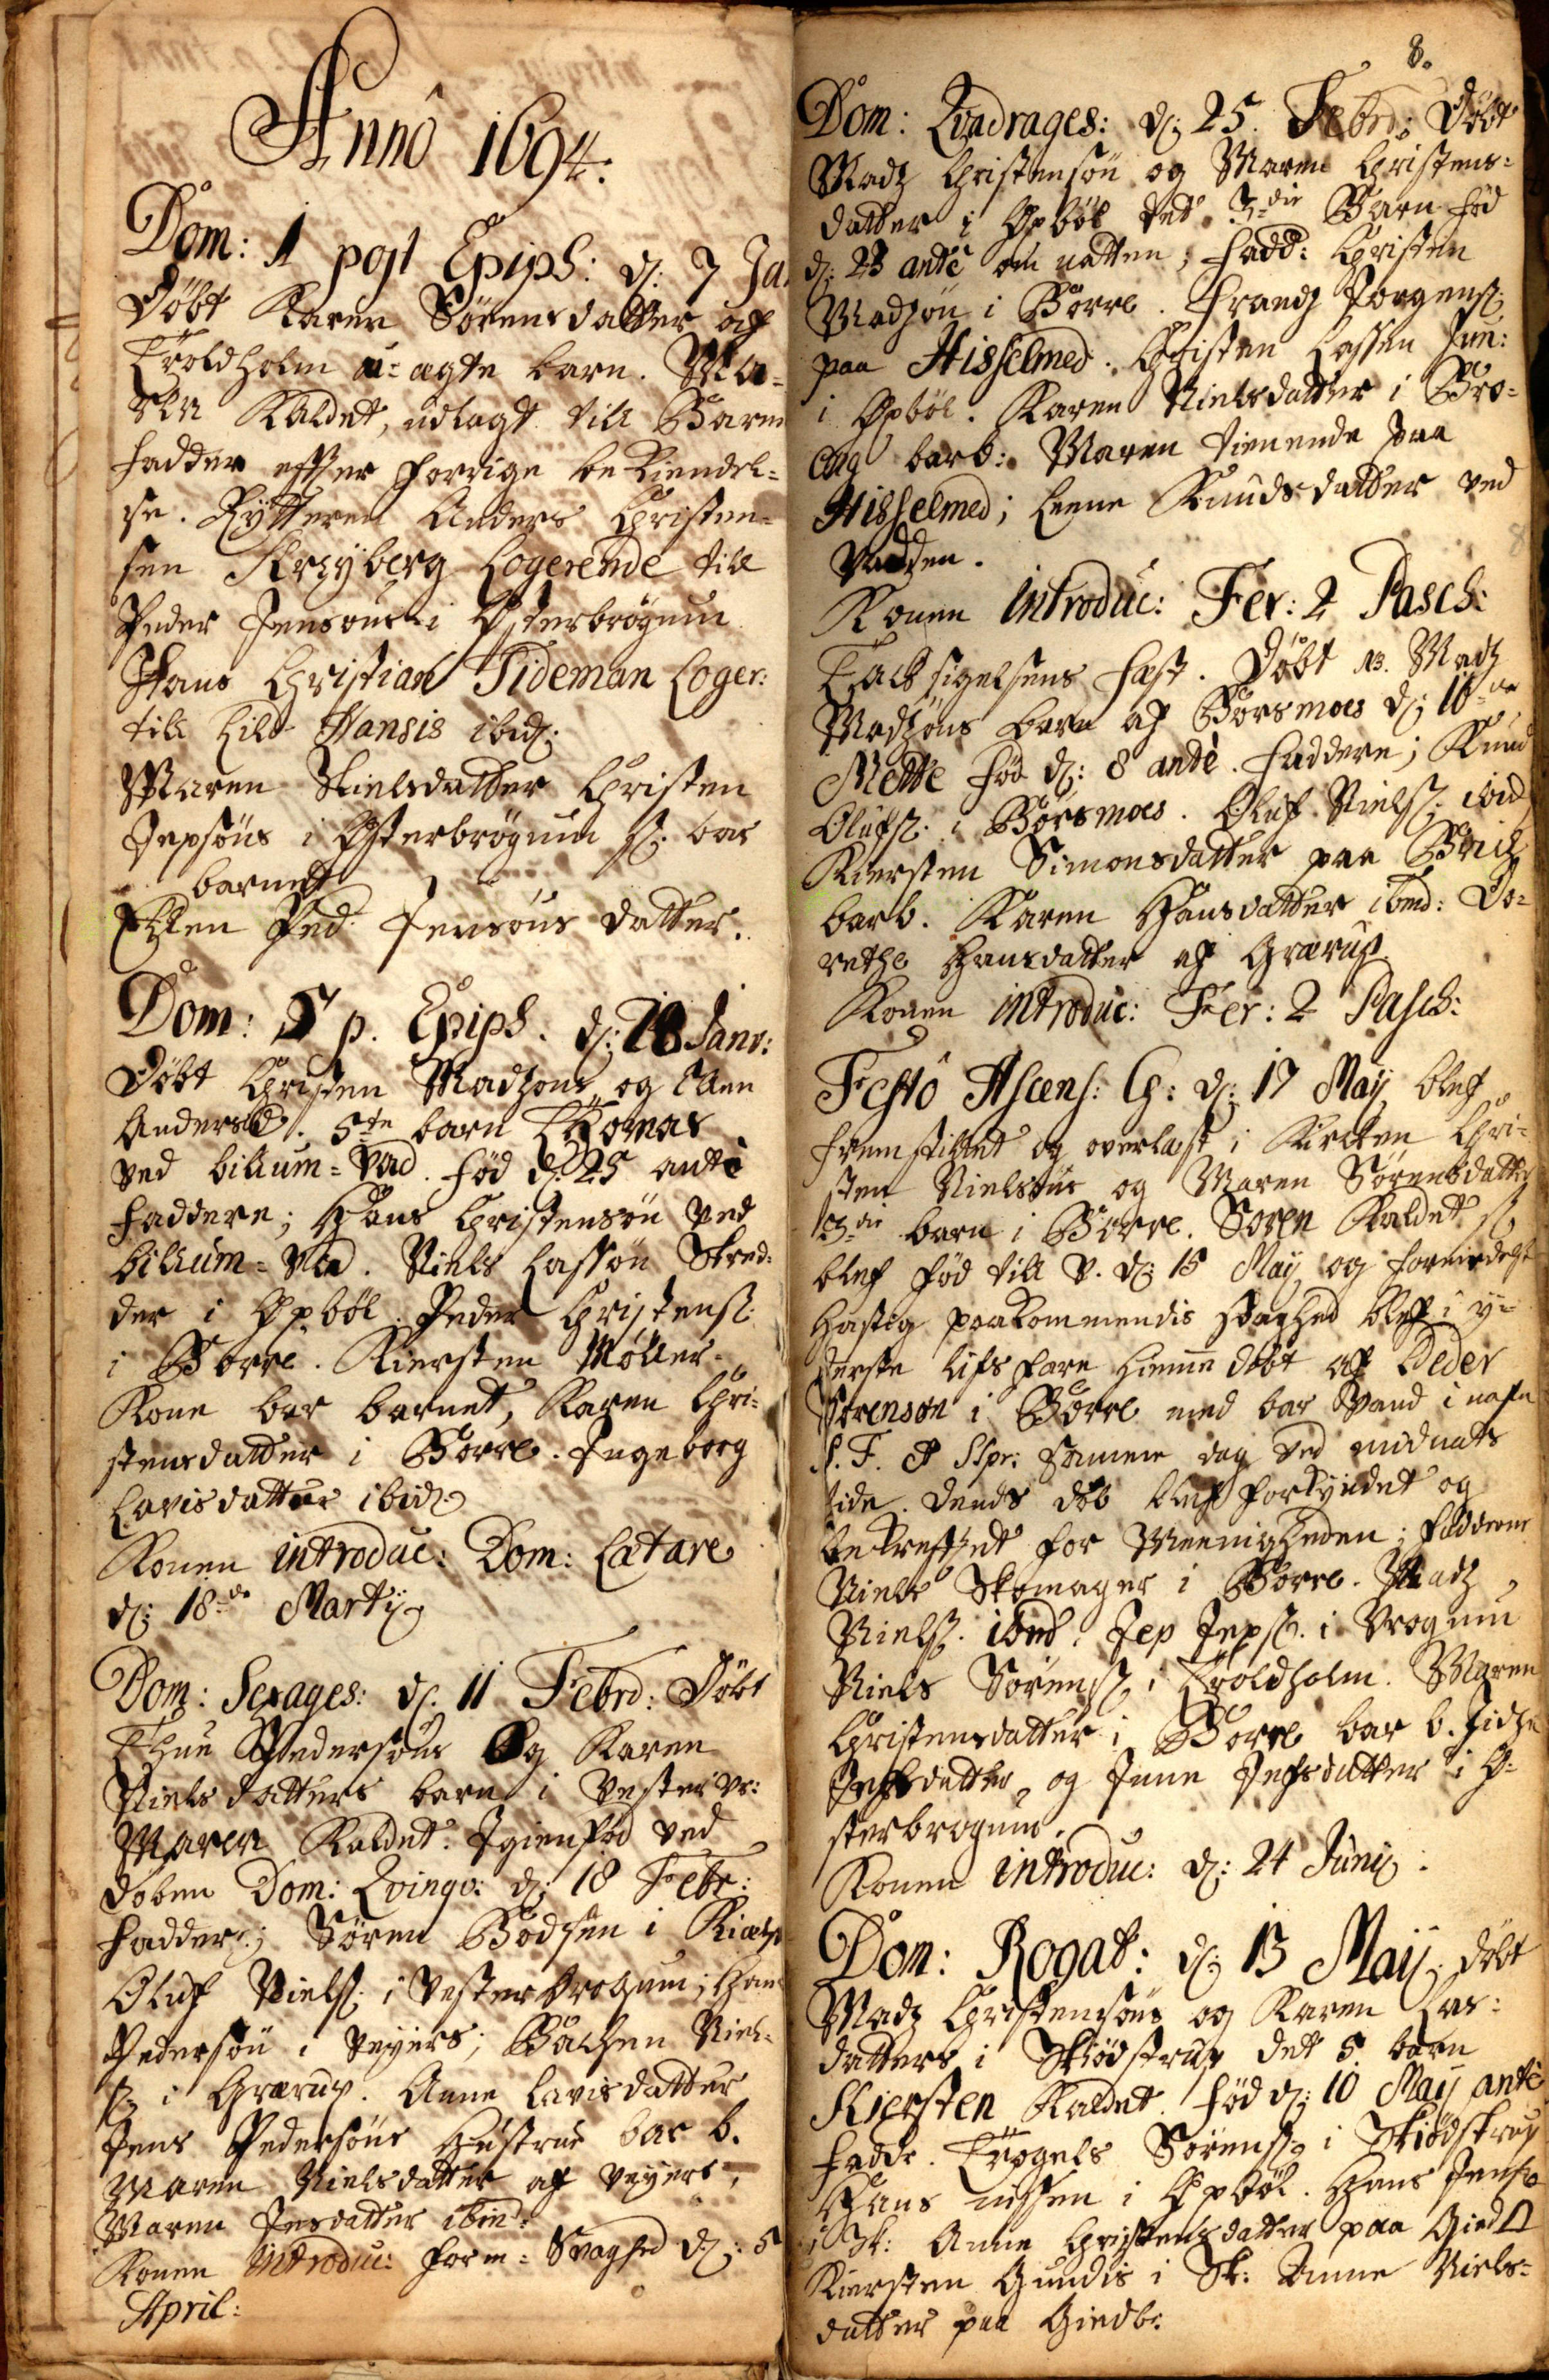Anno 1694:
Dom: 1 post Epiph: d: 7 Ja
Døbt Karen Sørens datter af
Troldholm u-ægte barn. Ma¬
ren kaldet, udlagt till Barne¬
Faddere effter forrige bekiendel¬
se. Rytteren Anders Christen¬
sen Kryberg Logerende till
Peder Jensøns i Østerbrøgum.
Hans Christian Tideman Loger:
til Lild Hansis ibid:
Maren Nielsdatter Christen
Jepsøns i Østerbrøgum s. bar
Barnet.
Ellen Ped: Jensøns datter.
Dom: 5 p. Epiph. d: 28 Janv:
Døbt Christen Madzøns og Ellen
Andersd. 5te barn Thomas
ved billum=vad fød d. 25 ante
Faddere; Hans Christensøn ved
billum=vad, Niels Lassøn Skred¬
der i Oxbøl, Peder Christens.
i Borre. Kiersten Møller¬
Kone bar barnet, Karen Chri¬
stensdatter i Borre . Ingeborg
Lavisdatter ibid.
Konen introduc: Dom: Latare
d: 18de Martij.
Dom: Sexages: d. 11 Febr: Døbt
Thue Pedersøns og Karen
Niels datters barn i Vester Vr:
Maren Kaldet: Igienfød ved
doben Dom: Qvinqv: d: 18 Febr:
Faddere: Søren Bodsen i Kiæ##
Oluf Niels: i Vester brogum; Hans
Pedersøn i Veijers; Bachen Niel¬
s i Grærup. Anne Lavisdatter
Jens Pedersøns Hustrue bar b.
Maren Nielsdatter af Veyers,
Maren Jesdatter ibid:
Konen introduc: for m=Svaghed d: 5
April:
8
Dom. Qvadrages: d 25. Febr: Døbt
Madz Christensøn og Maren Christens¬
datter i Oxbøl det 3die Barn fød
d: 23 ante om natten; Fadd: Christen
Madzøn i Borre, Frandz Jorgens.
paa Hisselmed. Christen Lassen Jun:
i Oxbøl. Karen Nielsdatter i Bro¬
eng barb: Maren tienende paa
Hisselmed; Leene Knudsdatter ved
Vadden.
Konen introduc: Fer. 2 Pasch:
Tacksigelsens Fæst. Døbt ##. Madz
Madzøns barn af Børsmoes d: 10de
Mette fød d: 8 ante. Faddere; Knud
Olufs. i Børsmoes. Oluf Niels. ibid.
Kiersten Simonsdatter paa Brich
barb. Karen Hansdatter ibm: Do¬
rethe Hansdatter af Grærup.
Konen introduc: Fer: 2 Pasch:
Festo Ascens: Ch: d: 17 May blef
fremstillet og overlæst i Kirchen Chri¬
sten Nielsøns og Maren Sørensdatters
3die Barn i Borre. Soren kaldet s
blef fød till V. d: 15 May og formiedelst
hastig paakommendis Svaghed blef i y¬
derste lifs fare hiemme døbt af Peder
Sørensøn i Borre med bar Vand i nafn
S.F. ## Sspr: samme dag ved midnats
tide. dends dob blef forkyndet og
bekreftet for Meenigheden; Fadderne
Niels Skomager i Borre, Madz
Niels. ibm. Jep Jeps. i Vrogum
Niels Sørens i Troldholm. Maren
Christensdatter i Borre bar b. Zidze
Jepsdatter og Jene Jepsdatter i Ø¬
sterbrogum.
Konen introduc: d: 24 Junij.
Dom: Rogat: d:13 Maij døbt
Madz Christensøns og Karen Las¬
datters i Skiødstrup det 5. barn
Kiersten kaldet. fød d: 10 Maij ante
Faddr. Trogels Sørens i Skiødstrup
Hans ##sen i Oxbøl. Hans Jens
i Sk: Anne Christensdatter paa Gied##
Kiersten Gundis i Sk: Anne Niels¬
datter paa Giedb.
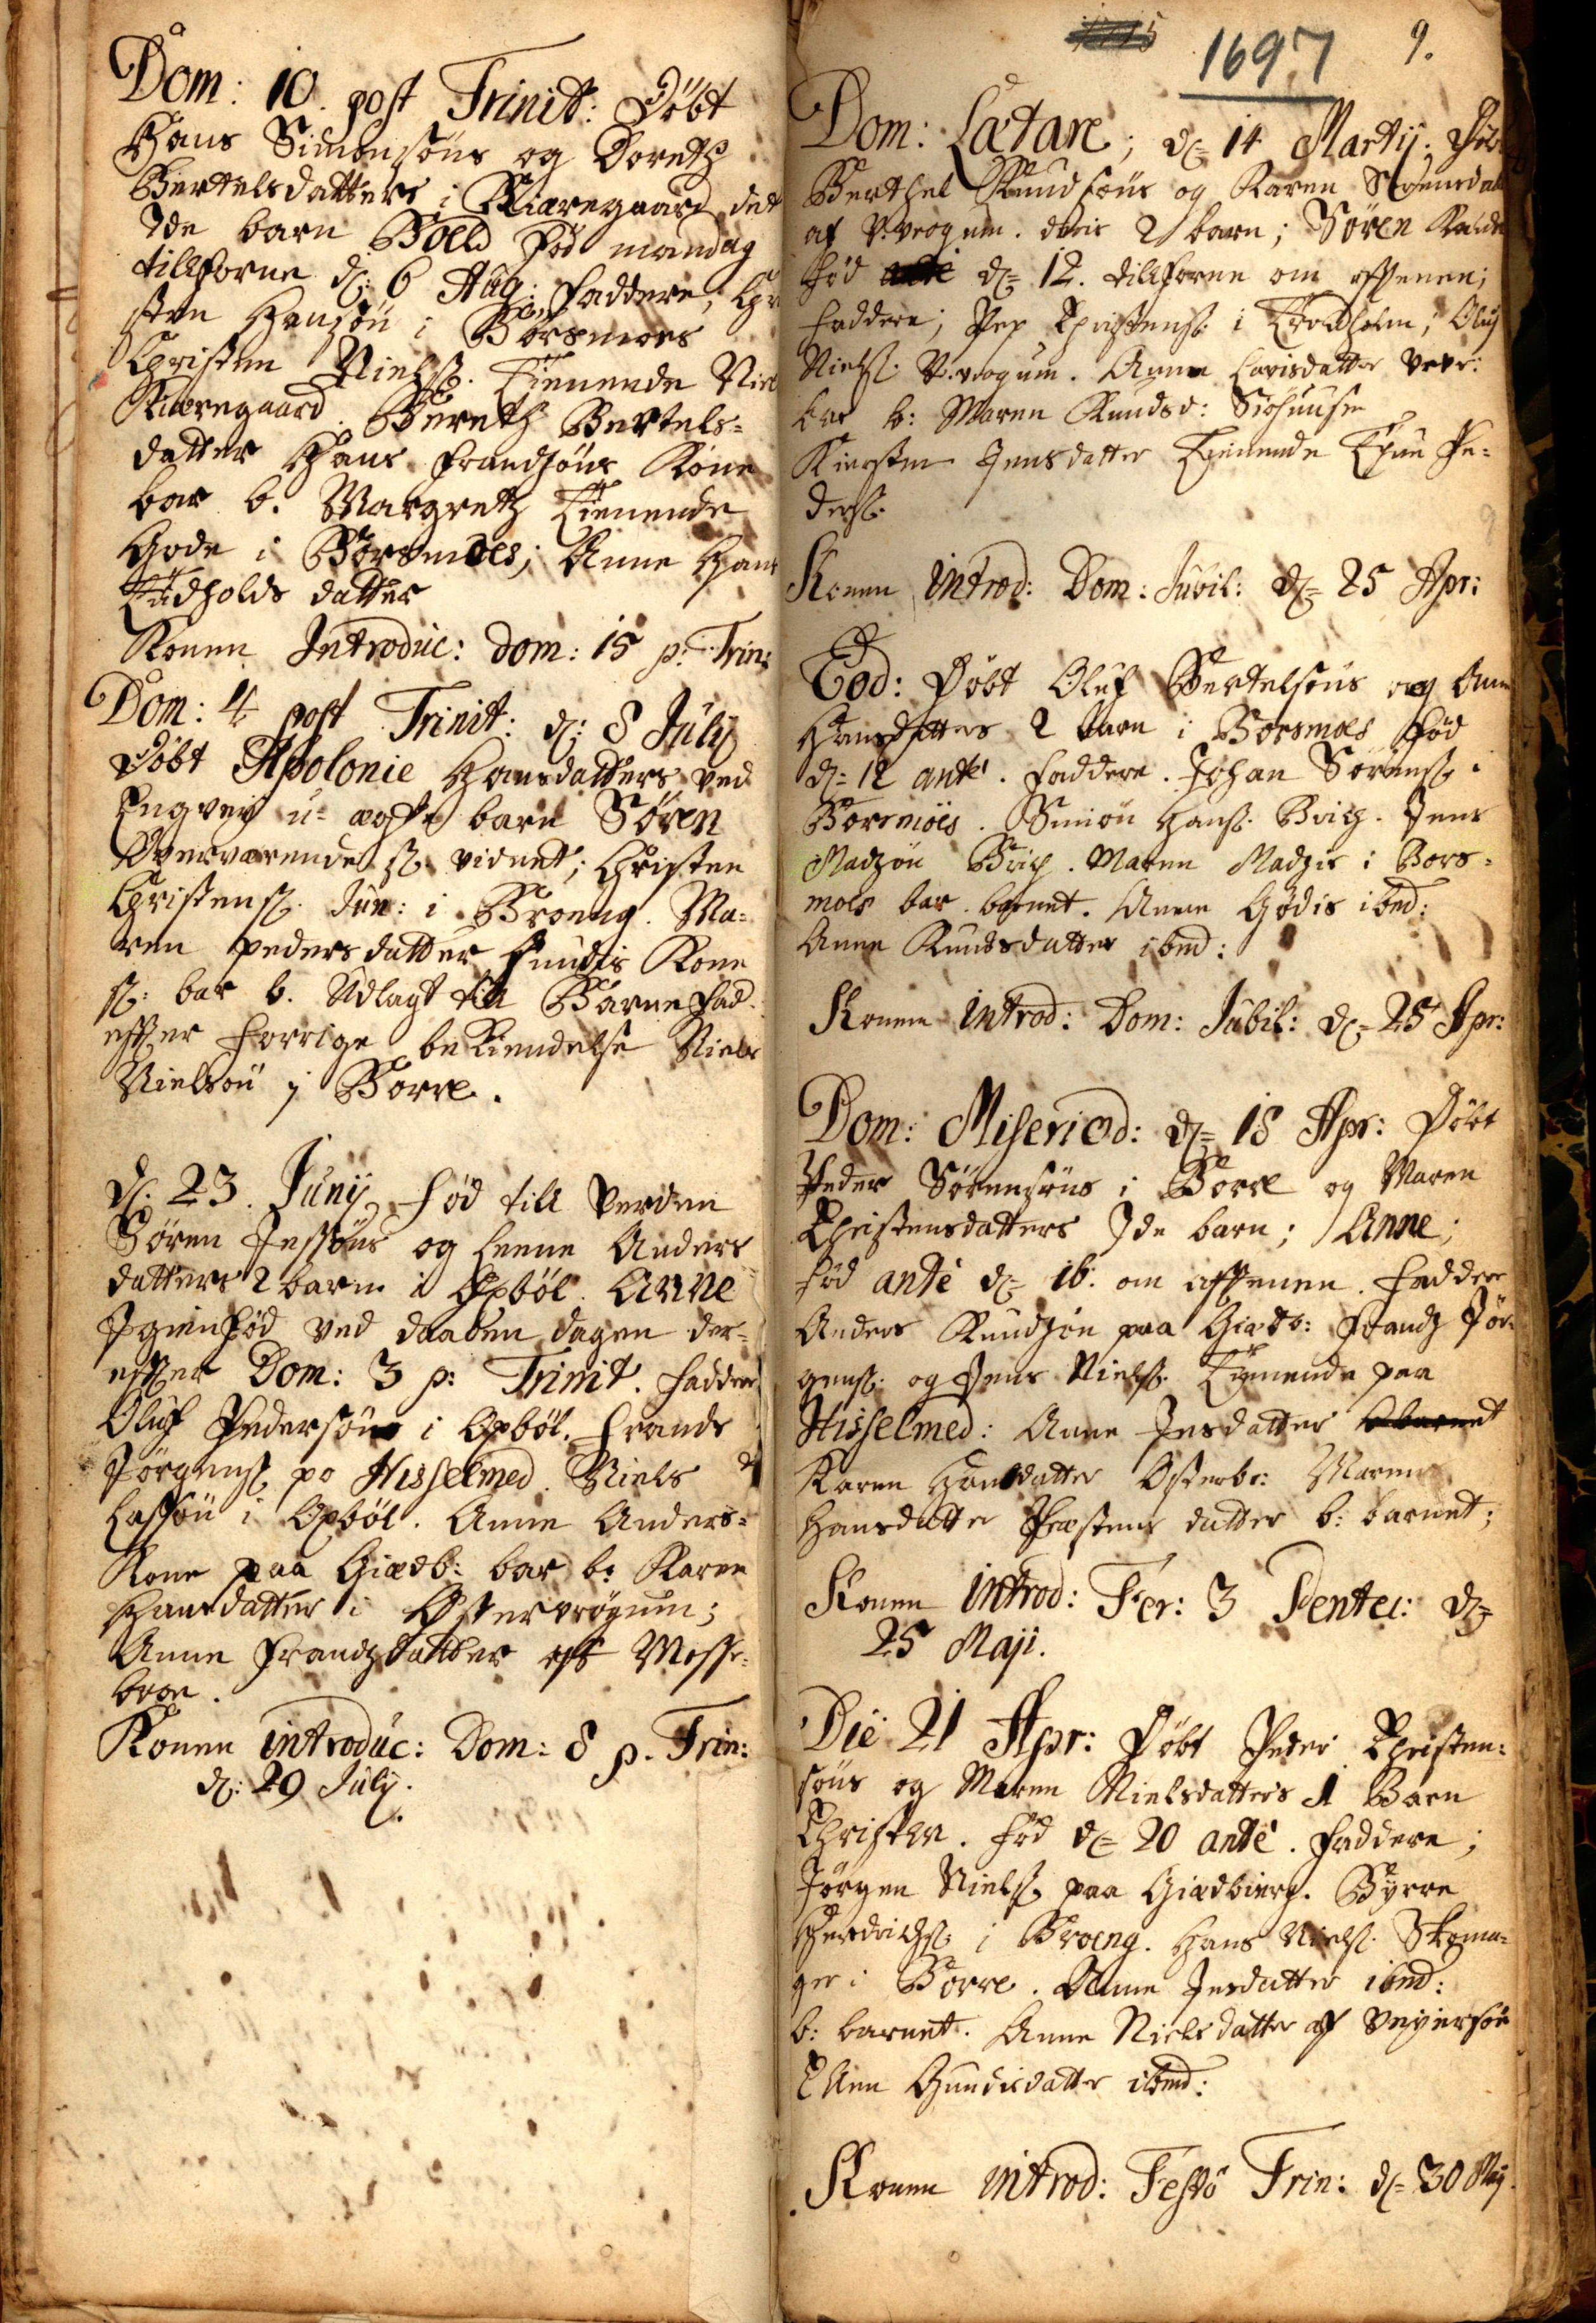Dom: 10. post Trinit: Døbt
Hans Simonsøns og Dorth
Bertelsdatters i Biæregaard det
7de barn Boeld fød mandag
tillforne d: 6 Aug: Faddere; Chr
sten Hansøn i Børsmoes
Christen Niels. Tienende Niels
Kiæregaard. Bereth Bertels¬
datter Hans Frandsøns Kone
bar b. Margreth Tienende
Gode i Børsmoes; Anne Hans
Tudholds datter
Konen Introduc: Dom: 15 p: Trin:
Dom: 4 post Trinit: d. 8 Julij
Døbt Apolonie Hansdatters ved
Engvey u=ægte barn Søren
Overværende s. vidnet; Christen
Christens. Jun: i Broeng. Ma¬
ren pedersdatter ##undis Kone
s: bar b. Udlagt till Barnefad.
effter forrige bekiendelse Niels
Nielsøn i Borre.
D. 23. Junij fød till Verden
Søren Jessøns og Leene Anders
datters 2 barn i Oxbøl. Anne
Igienfød ved daaben dagen der¬
effter Dom: 3 p: Trinit. Faddere
Oluf Pedersøn i Oxbøl, Frands
Jørgens po Hisselmed. Niels
Lassøn i Oxbøl. Anne Anders¬
Kone paa Giædb: bar b., Karen
Hansdatter i Østervrøgum;
Anne Frandzdatter aff Mosse¬
broe.
Konen introduc: Dom: 8 p. Trin:
d. 29 Julij.
9
1697
Dom: Latare; d. 14 Martij; Døbt
Berthel Knudsøns og Karen Sorensdat
af v. vrøgum. deris 2 barn; Søren kalde
fød ## d= 12. tillforne om aftenen;
Faddere; Jep Christens. i Troldholm; Oluf
Niels. V.vrogum. Anne Lavisdatter Vrvr:
bar b., Maren Knudsd: Siøhuuse
Kiersten Jensdatter Tienende Thue Pe¬
ders.
Konen introd: Dom: Jubil: d= 25 Apr:
Eod: Døbt Oluf Bertelsøns og Anne
Hansdatters 2 barn i Børsmoes fød
d=12 ante. Faddere. Johan Sørens. i
Børsmoes. Simon Hans. Brich. Jens
Madzøn Brich. Maren Madzes i Bors¬
moes bar barnet. Anne Gødis ibm:
Anne Knudsdatter ibm:
Konen introd: Dom: Jubil: d= 25 Apr:
Dom. Misericord. d.18 Apr: Døbt
Peder Sørensøns i Borre og Maren
Christensdatters 7de barn; Anne;
fød ante d= 16: om aftenen. Faddere
Anders Knudzøn paa Giædb: Frandz Jør¬
gens. og Jens Niels. Tienende paa
Hisselmed: Anne Jesdatter ##
Karen Hansdatter Østerbr: Maren
Hansdatter Præstens datter b. barnet;
Konen introd: Fer: 3 Pentec: d.
25 Maji.
Die 21 Apr: Døbt Peder Christen¬
søns og Maren Nielsdatters 1 Barn
Christen. fød d= 20 ante. Faddere;
Jørgen Niels paa Giædbierg, Byrre
Hendrichs i Broeng. Hans Niels. Skoma¬
ger i Borre. Anne Jesdatter ibm:
b: barnet. Anne Nielsdatter af Veyersøe,
Ellen Gundesdatter ibm:
Konen introd: Festo Trin: d= 30 Maij.